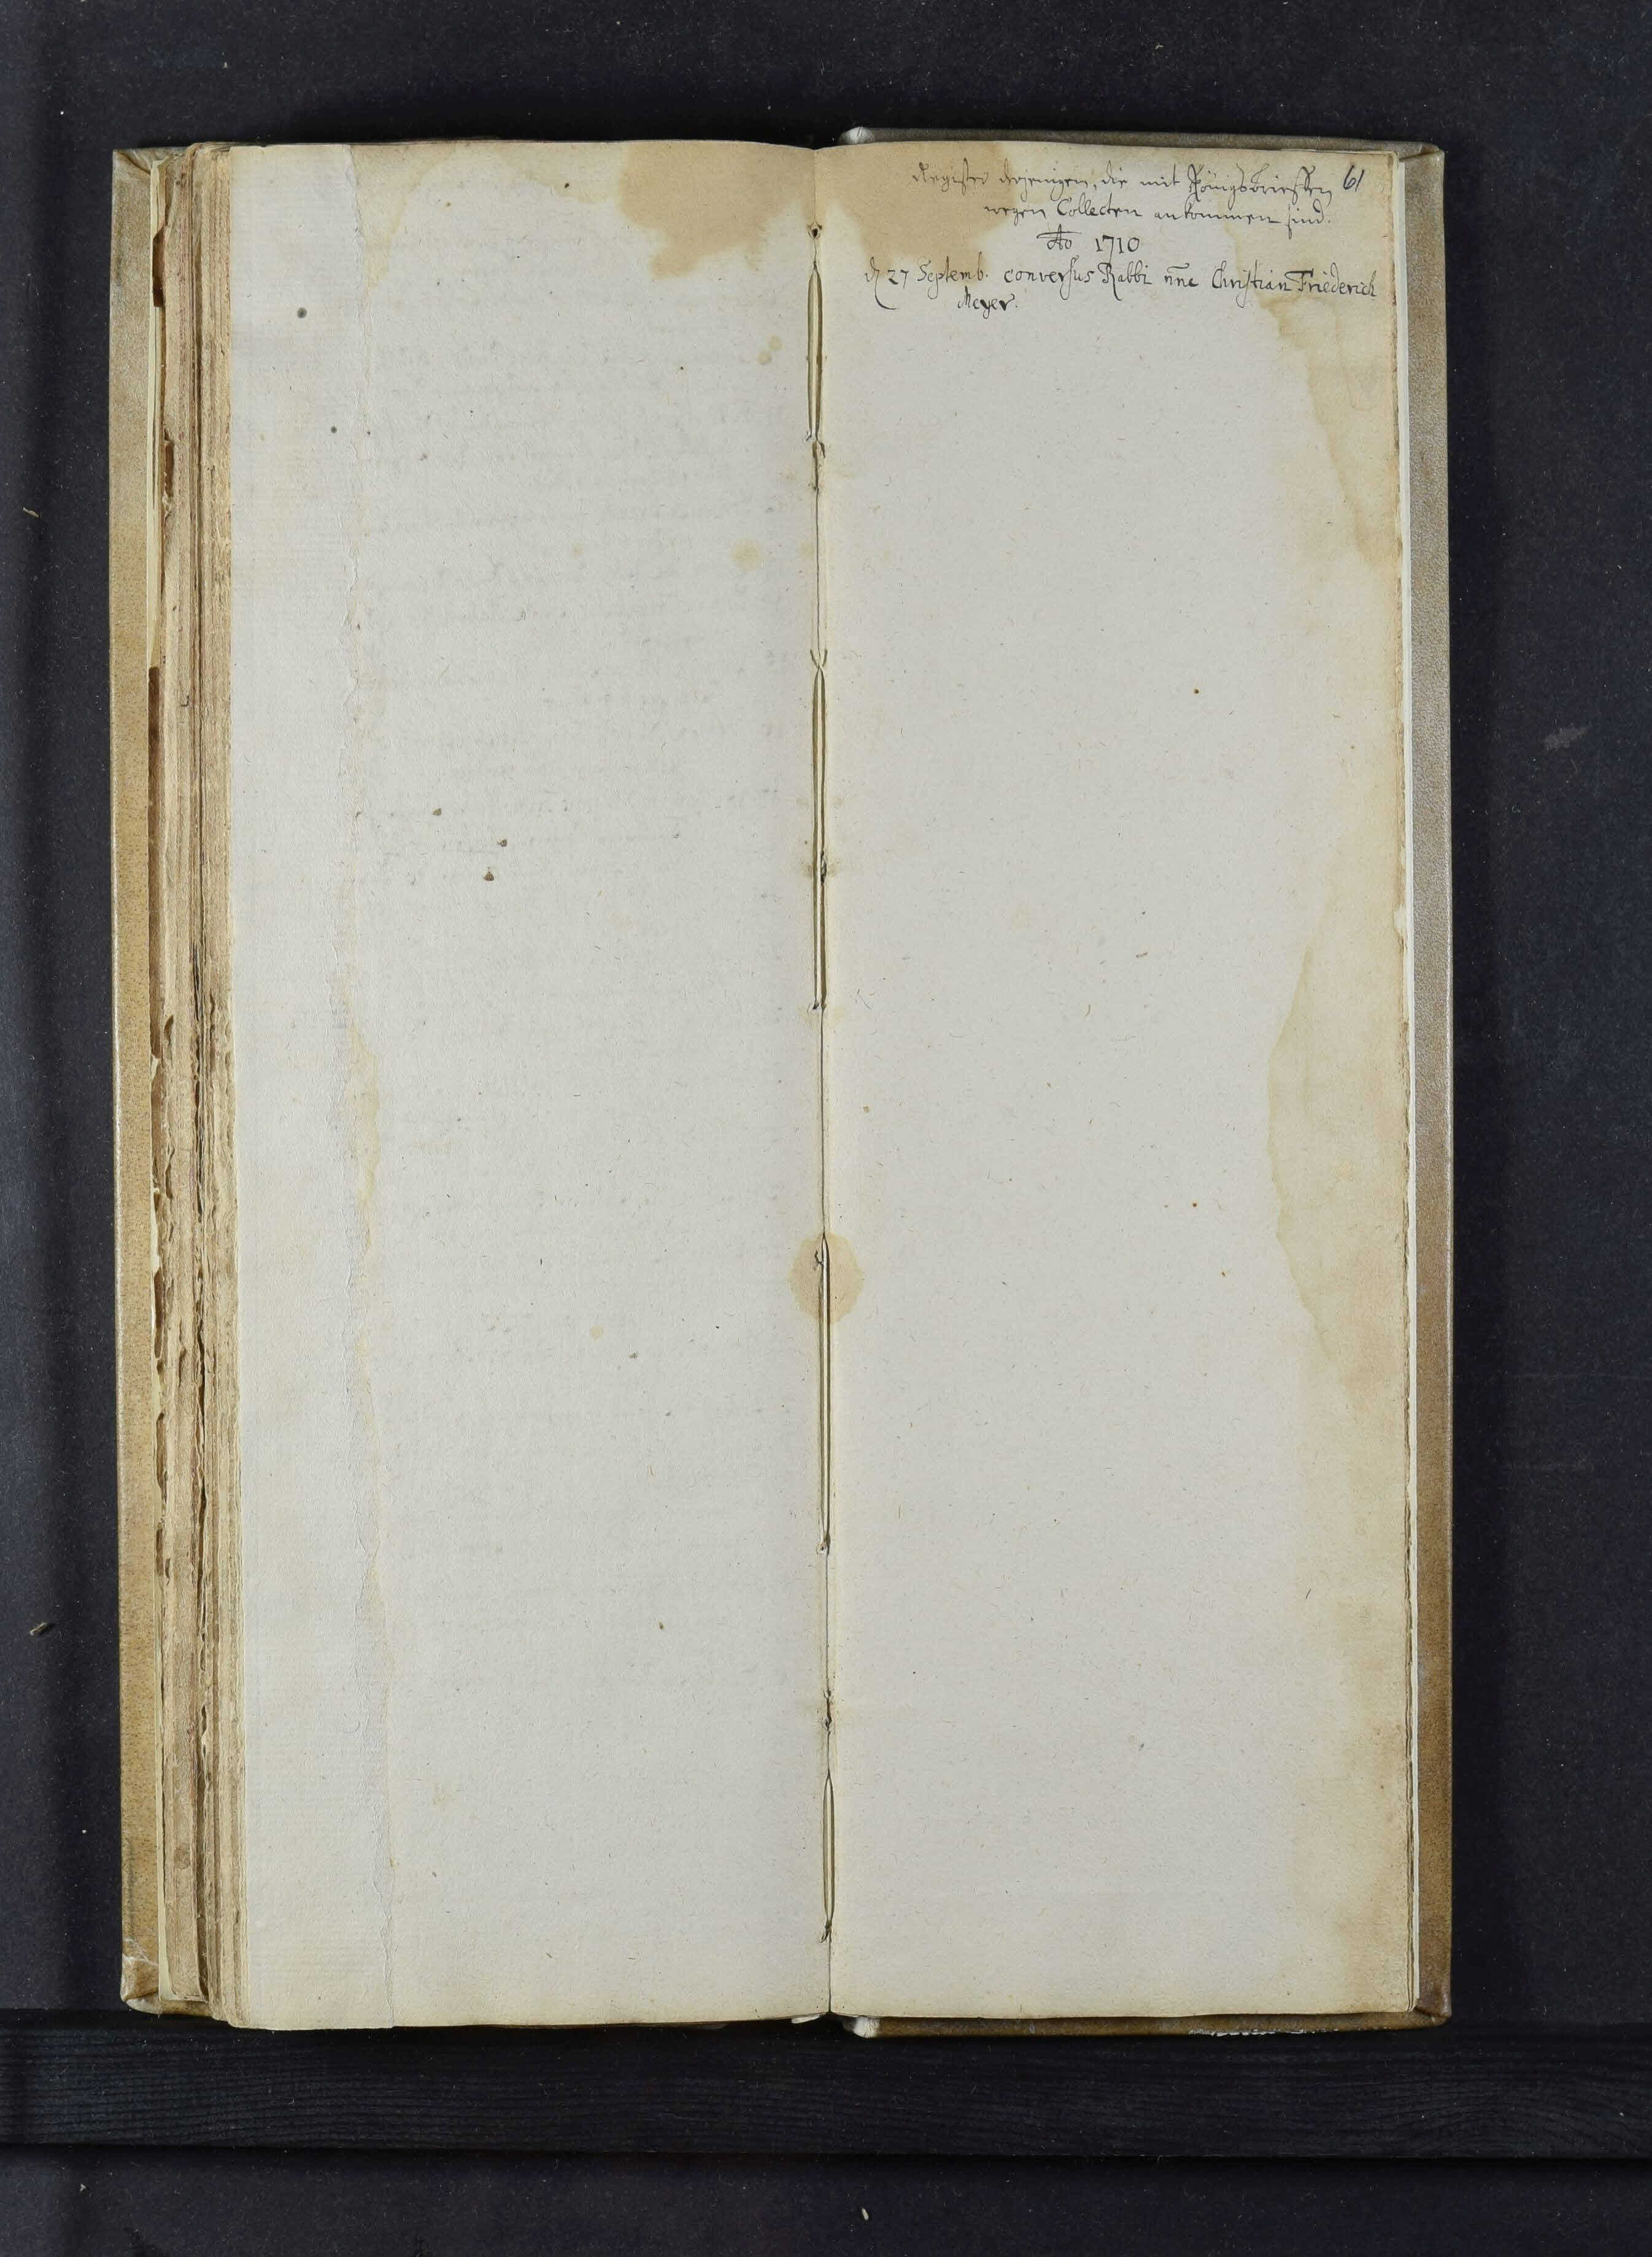61
Register devjenigen, dir mit Kønigs Brieffen
##egen Collecten ankommen sind.
Ao 1710
d 27 Septemb. conversus Rabbi ##nc Christian Friederich
Meyer.